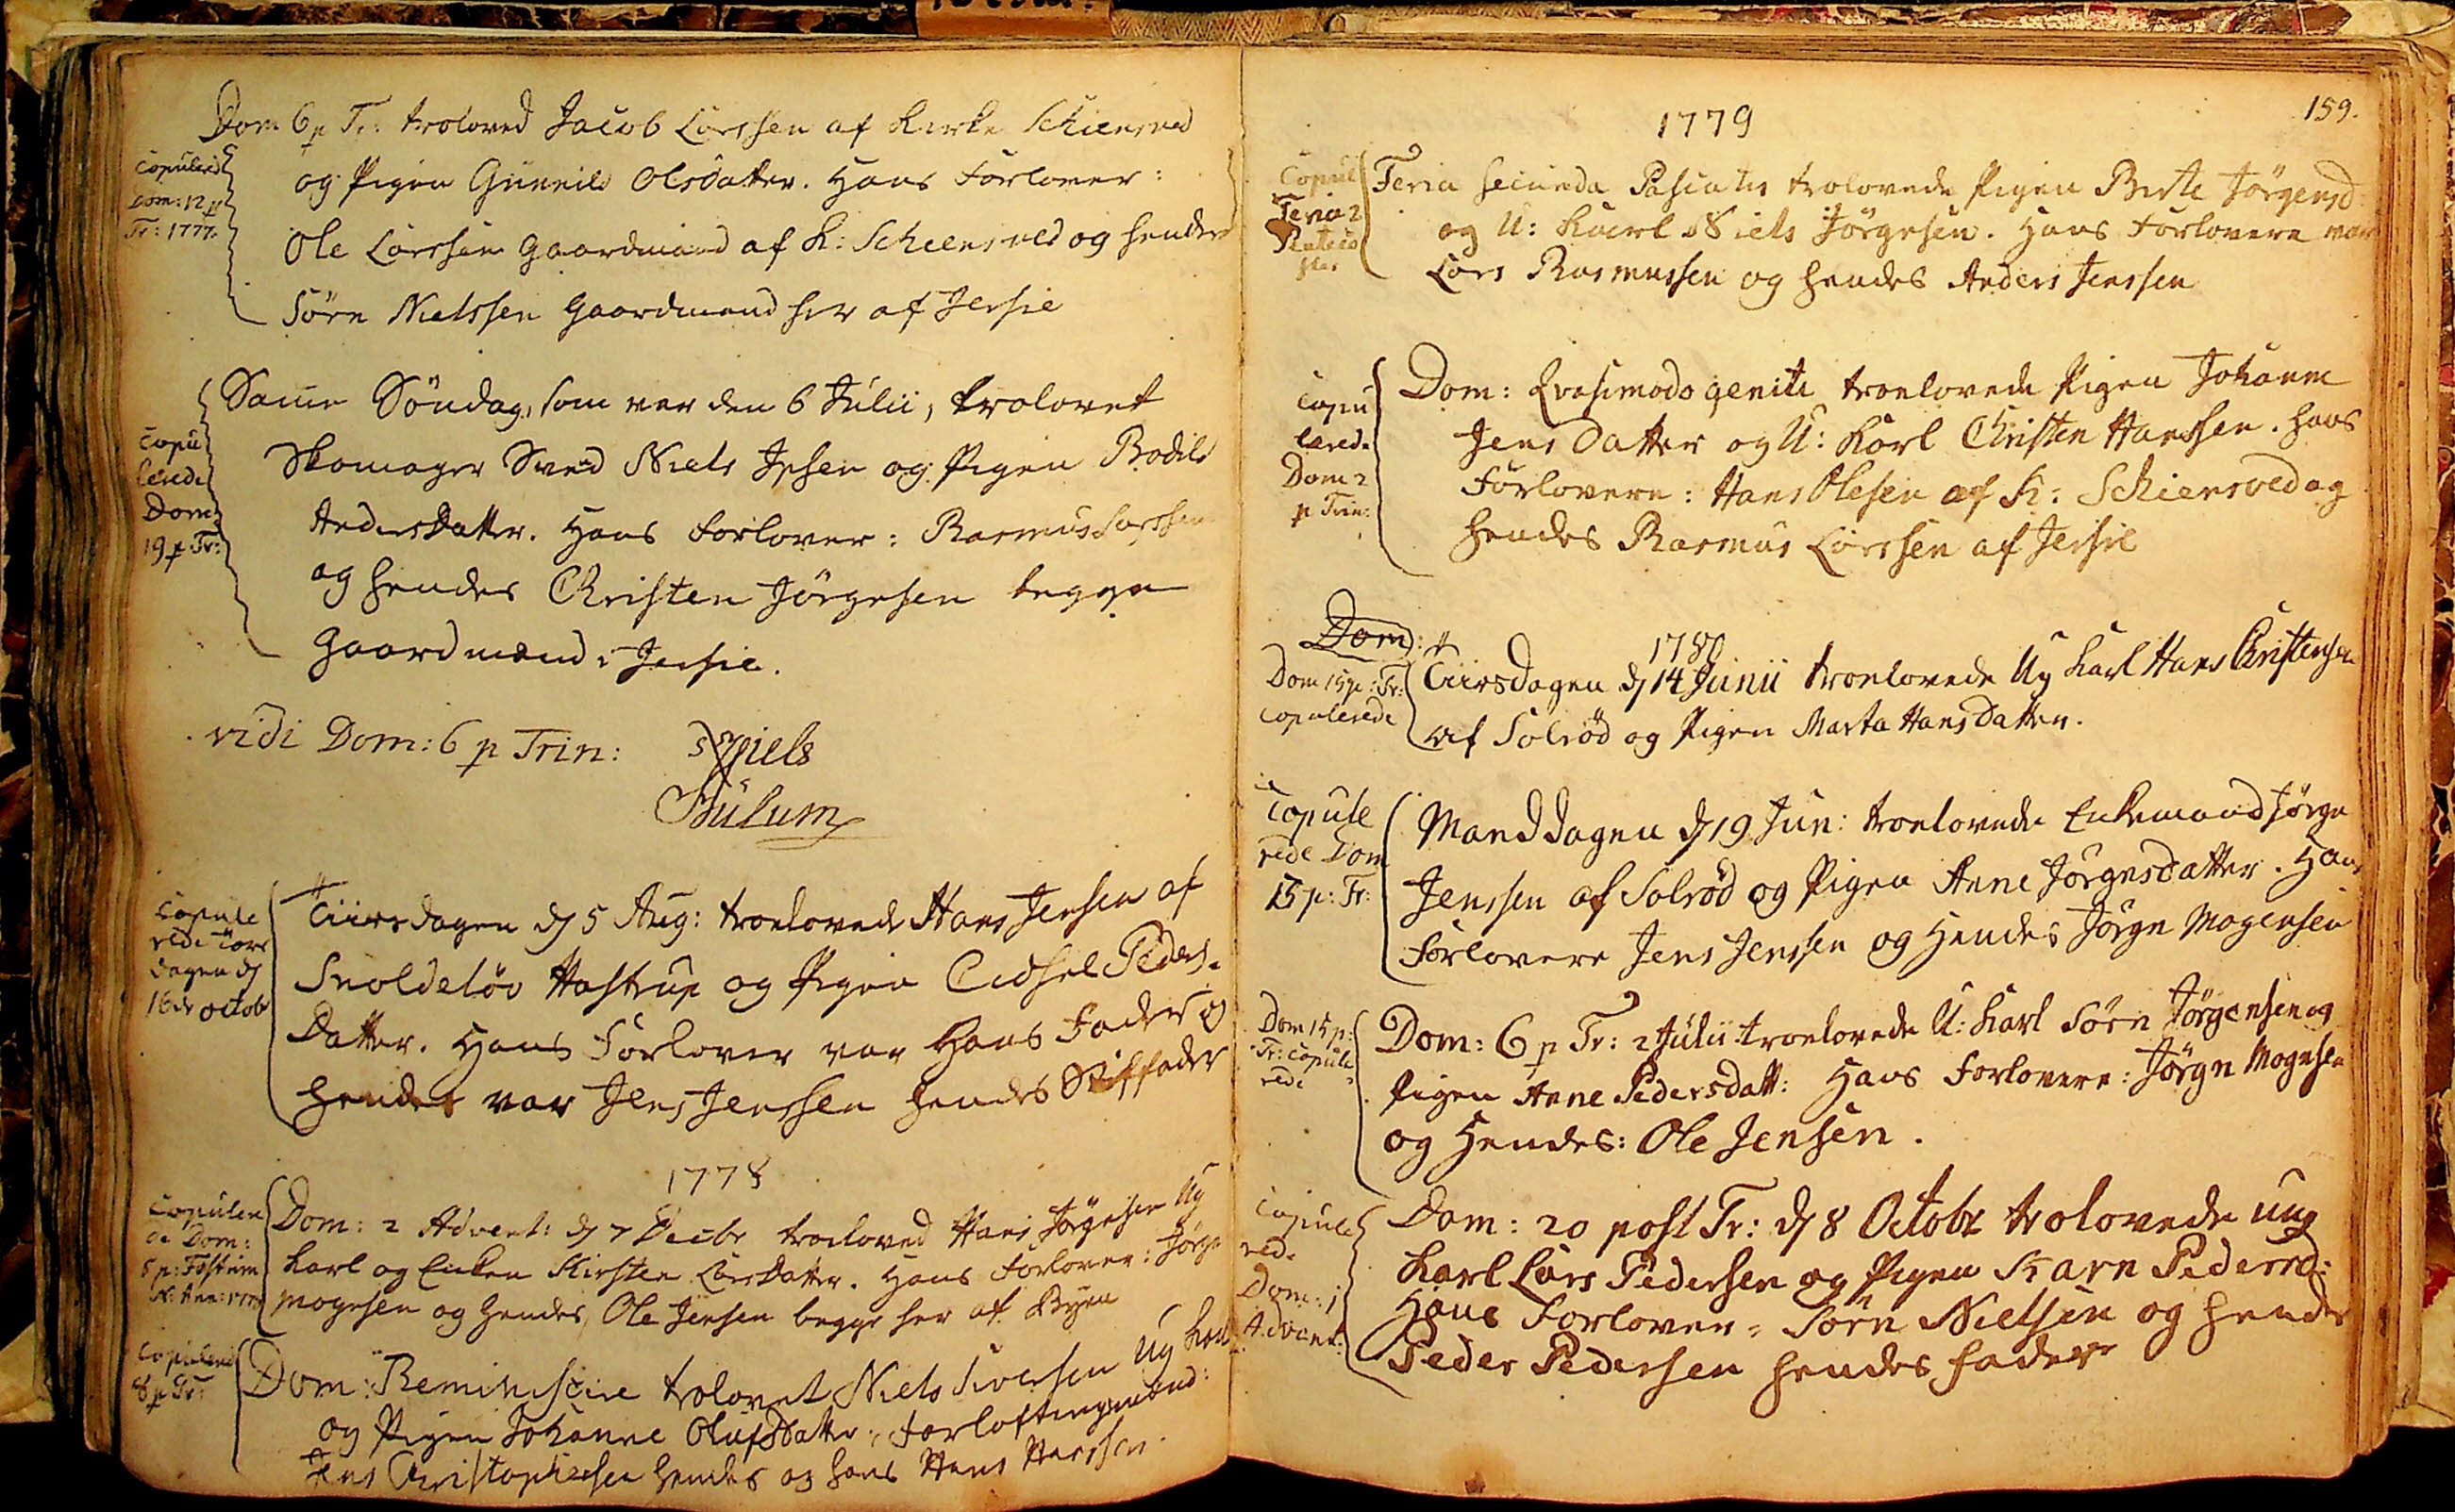Dom 6 p Tr: troloved Jacob Larsen af Kirke Schiensved
og Pigen Gunnild Olsdatter. Hans Forlover:
Ole Larssen Gaardmand af K: Schiensved og hendes
Sørn Nielssen Gaardmand her af Jersie
Copulered
Dom: 12 p
Tr: 1777:
Samme Søndag, som var d 6 Julii, trolovet
Skomager Svend Niels Ipsen og Pigen Bodild
AndersDatter. Hans Forlover: Rasmus Larssen
og hendes Christen Jørgnsen begge
Gaardmænd i Jersie
Copu
lerede
Dom:
19 p. Tr:
Vidi Dom: 6 p Trin: Niels
Aulum
Tiirsdagen d 5 Aug: Troeloved Hans Jensen af
Snoldeløv Hastrup og Pigen Cidsel Peders¬
Datter. Hans Forlover var Hans Fader og
hendes var Jens Jensen hendes Stiffader
Copule
rede Tors
dagen d
16de octobr
1778
Dom: 2 Advent: d 7 Decbr troeloved Hans Jørgensen Ug
karl og Enken Kirsten LarsDatter. Hans Forlover: Jørgn
Mognsen og hendes Ole Jensen begge her af Byen
Copulere
de Dom:
5 p: Festum
N:Ann: 1778
Dom: Reminiscere trolovet Niels Sivesen Ug karl
og Pigen Johanne OlufsDatter., forloftingsmænd:
Jens Christophersen hendes og hans Hans Hansen.
Copulered
8 p Tr:
159.
1779
Feria secunda Pascates trolovede Pigen Birte Jørgensd:
og U: Karl Niels Jørgnsen. Hans Forlovere var
Lars Rasmussen og hendes Anders Jenssen
Copul
Feria 2
Penteco
stes
Dom Qvasimodo geniti troelovede Pigen Johanne
Jensdatter og U:karl Christen Hanssen. hans
Forlovere: Hans Olesen af K: Schiensved og
hendes Rasmus Larssen af Jersie
Copu¬
lerede
Dom 2
p Trin:
1780.
Tiirsdagen d 14 Junii troelovede Ugkarl Hans Christensen
af Solrød og Pigen Marta Hansdatter.
Dom:
Dom 15 p: Tr:
Copulerede
Manddagen d 19 Jun: troelovede Enkemand Jørgn
Jensen af Solrød og Pigen Anne Jørgnsdatter. Hans
Forlovere Jens Jenssen og Hendes Jørn Mogensen
Copule
rede Dom
15 p: Tr:
Dom: 6 p Tr: 2 Julii troelovede U:karl Sørn Jørgensen og
Pigen Anne Pedersdatt: Hans forlovere: Jørgn Mognsen
og Hendes: Ole Jensen.
Dom 15 p:
Tr: Copule
rede
Dom: 20 post Tr: d 8 Octobr trolovede ung
karl Lars Pedersen og Pigen Karn Pedersd:
Hans forlovere = Sørn Nielsen og hendes
Peder Pedersen hendes fader
Copule
rede
Dom: 1
Advent:
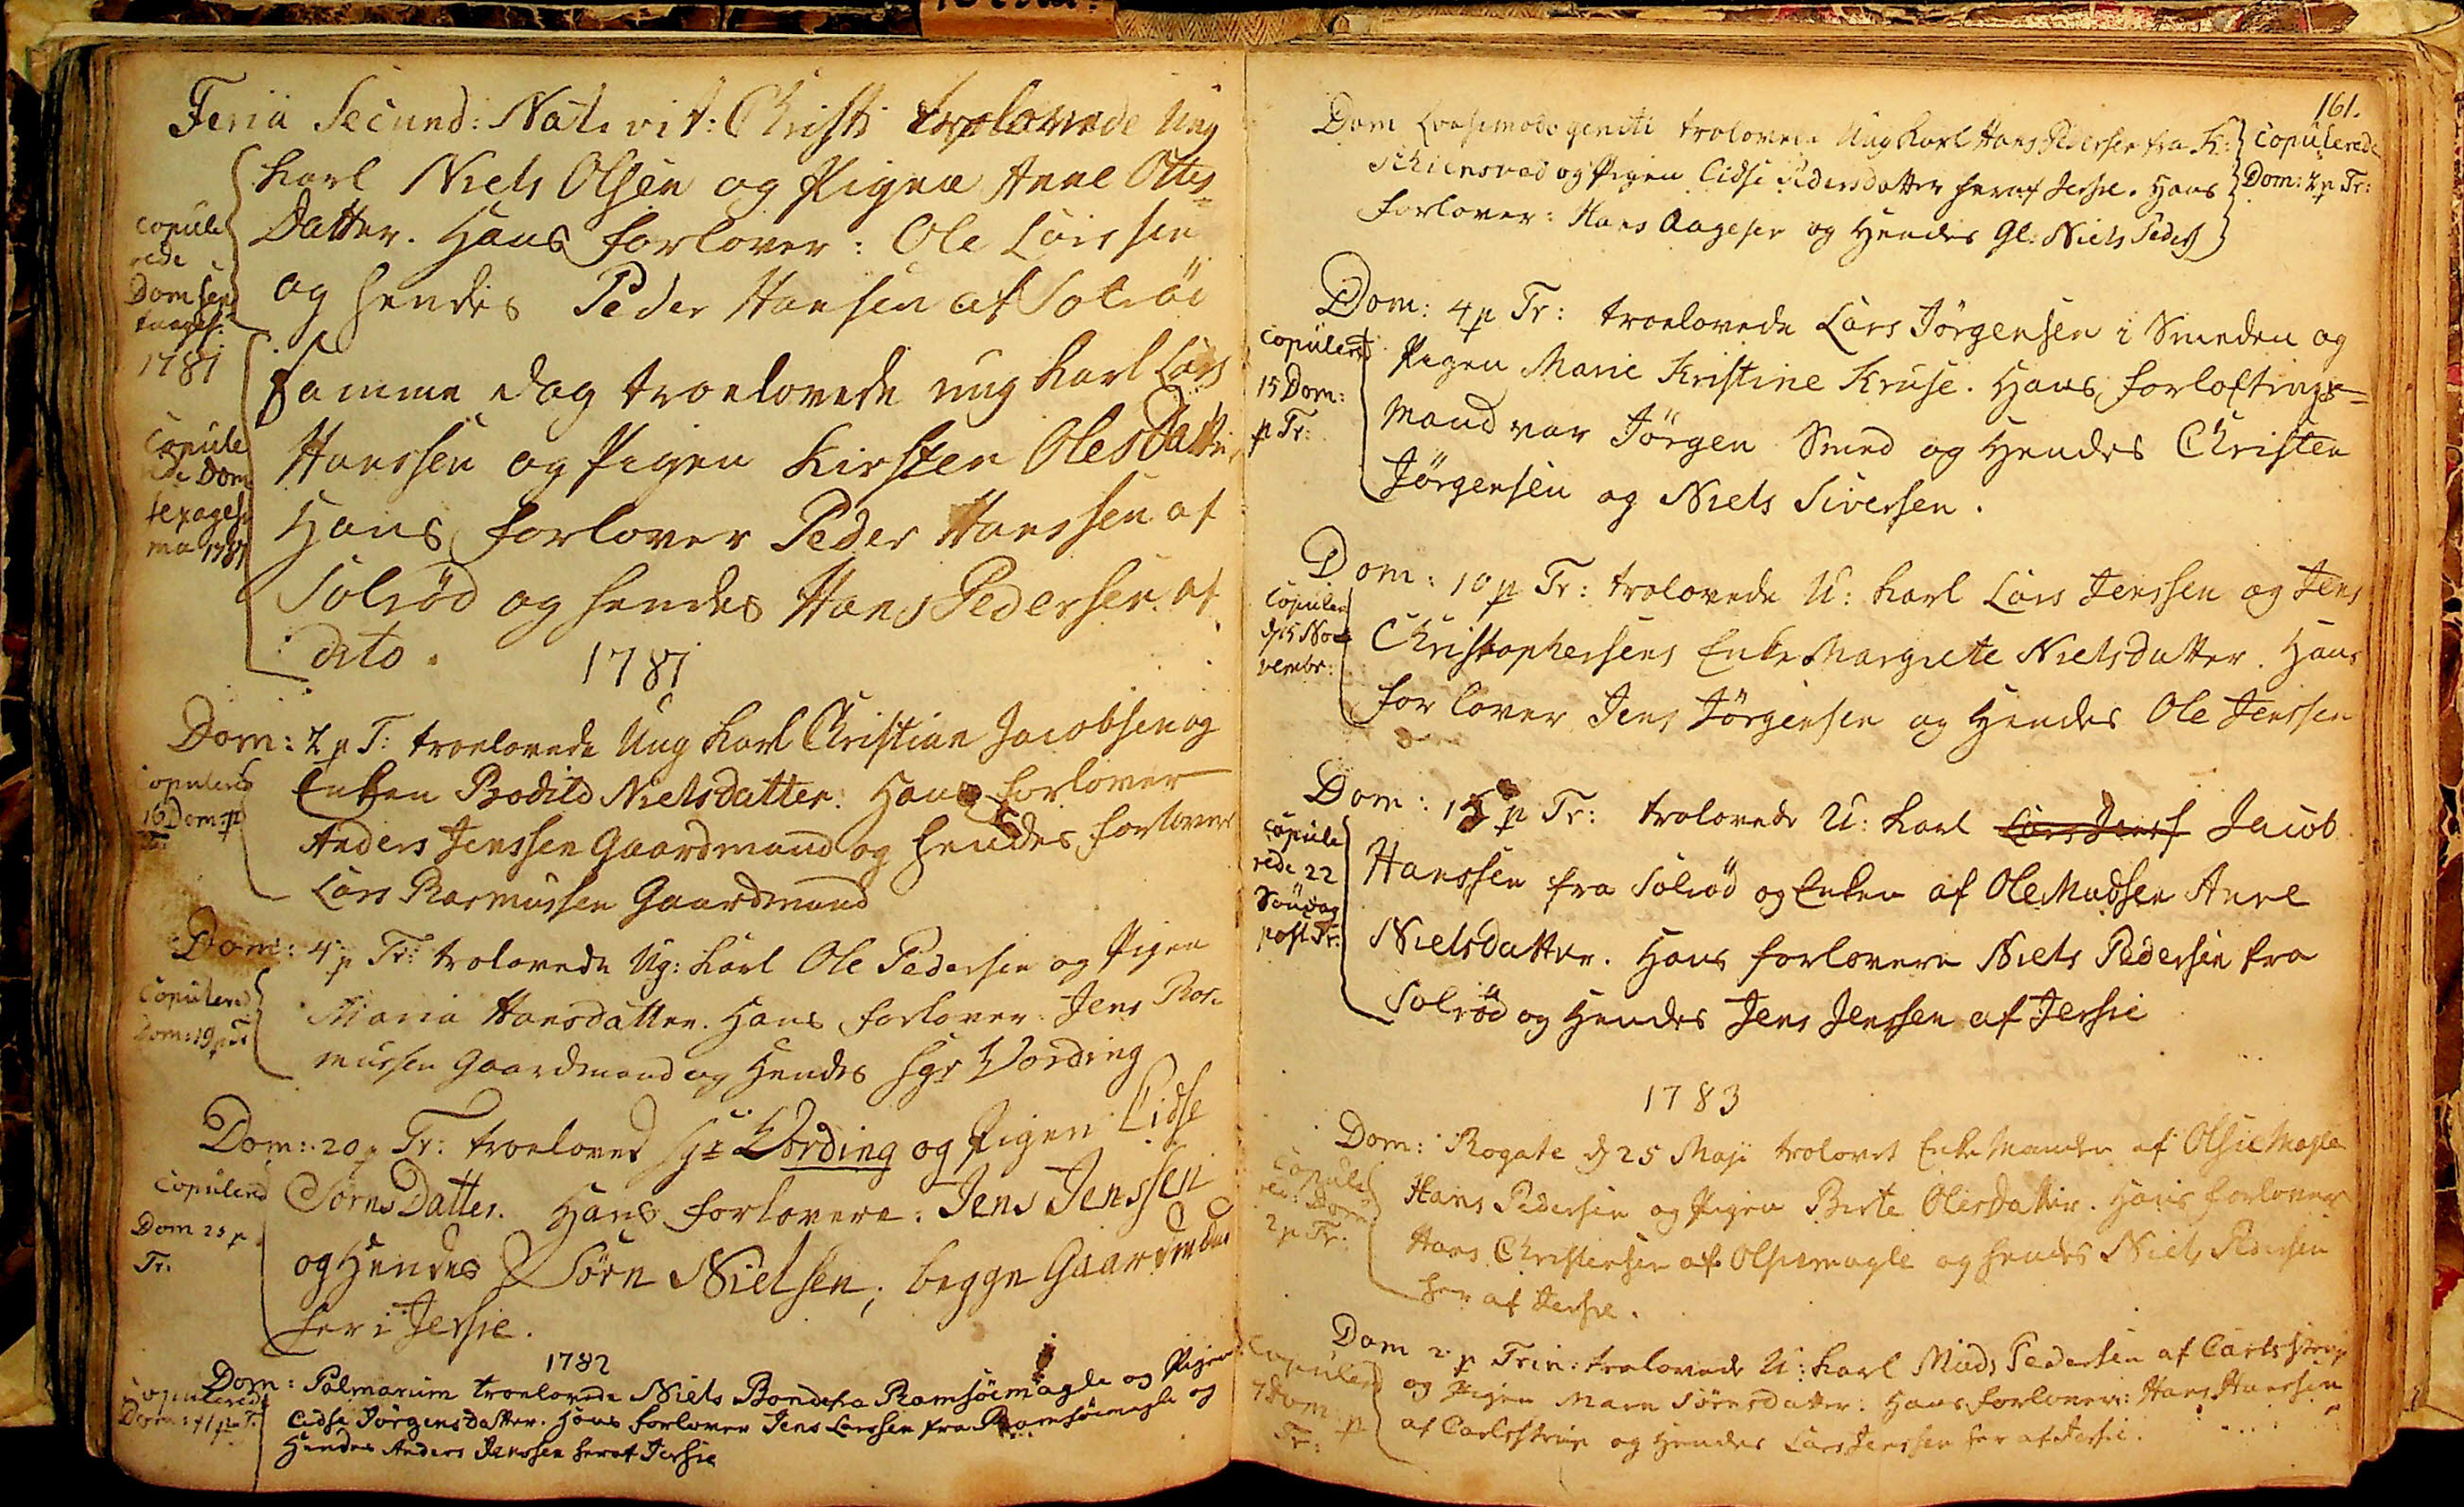Feria secund: Nativit: Christi trolovede Ung
karl Niels Olsen og Pigen Ane Ottes¬
Datter. Hans forlover: Ole Larssen
og hendes Peder Hansen af Solrød
Copule¬
rede
Dom sep
tuages:
1781
Samme dag troelovede ung karl Lars
Hanssen og Pigen Kirsten Olesdatter,
Hans forlover Peder Hanssen af
Solrød og hendes Hans Pedersen af
dito.
Copule
rede Dom
Sexagesi
ma 1781
1781
Dom: 2 p T: troelovede Ung Karl Christian Jacobsen og
Enken Bodild Nielsdatter. Hans Forlover
Anders Jensen Gaardmand og hendes forlover
Lars Rasmussen Gaardmand
Copulered
16 Dom. p
Tr:
Dom: 4 p Tr: trolovede Ug:Karl Ole Pedersen og Pigen
Maria Hansdatter. Hans forlover: Jens Ras¬
mussen Gaardmand og Hendes Sgr Vording
Copulered
Dom: 19 p Tr
Dom: 20 p Tr: troeloved Sgr Vording og Pigen Cidse
Sørns Datter. Hans forlovere: Jens Jenssen
og Hendes Sørn Nielsen; begge Gaardmænd
her i Jersie.
Copulered
Dom 25 p.
Tr:
1782
Dom: Palmarum troelovede Niels Bonde fra Ramsøemagle og Pigen
Cidse Jørgensdatter. Hans forlover Jens Larsen fra Ramsøemagle og
Hendes Anders Jensen heraf Jersie
Copulered
Dom:11p Tr
161.
Dom Qvasimodo geniti trolovede Ungkarl Hans Pedersen fra K:
Schiensved og Pigen Cidse Pedersdatter heraf Jersie. Hans
forlover: Hans Aagesen og Hendes Gl: Niels Peders
Copulerede
Dom: 2 p Tr:
Dom: 4 p Tr: troelovede Lars Jørgensen i Smeden og
Pigen Marie Kristine Kruse. Hans forloftings¬
Mand var Jørgen Smed og Hendes Christen
Jørgensen og Niels Siversen
Copulered
15 Dom:
p Tr:
Dom: 10 p Tr: trolovede U:karl Lars Jenssen og Jens
Christophersens Enke Margrete Nielsdatter. Hans
forlover Jens Jørgensen og Hendes Ole Jensen
Copuler:
d 15 No
vembr:
Dom: 15 p Tr: trolovede U:karl Lars Jenss Jacob
Hanssen fra Solrød og Enken af Ole Madsen Anne
Nielsdatter. Hans forlovere Niels Pedersen fra
Solrød og Hendes Jens Jenssen af Jersie
Copule¬
rede 22
Søndag
post Tr:
1783
Dom: Rogate d 25 Maji trolovet Enke Manden af ØlsieMagle
Hans Pedersen og Pigen Birte OlesDatter. Hans forlover
Hans Christensen af Ølsiemagle og hendes Niels Pedersen
her af Jersie.
Copule
red: Dom:
2 p Tr:
Dom 2. p Trin: trolovede U:karl Mads Pedersen af Carlstrup
og Pigen Marn Sørnsdatter: Hans forlovere: Hans Hansen
af Carlstrup og Hendes Lars Jenssen her af Jersie.
Coulered
7 Dom: p
Tr: